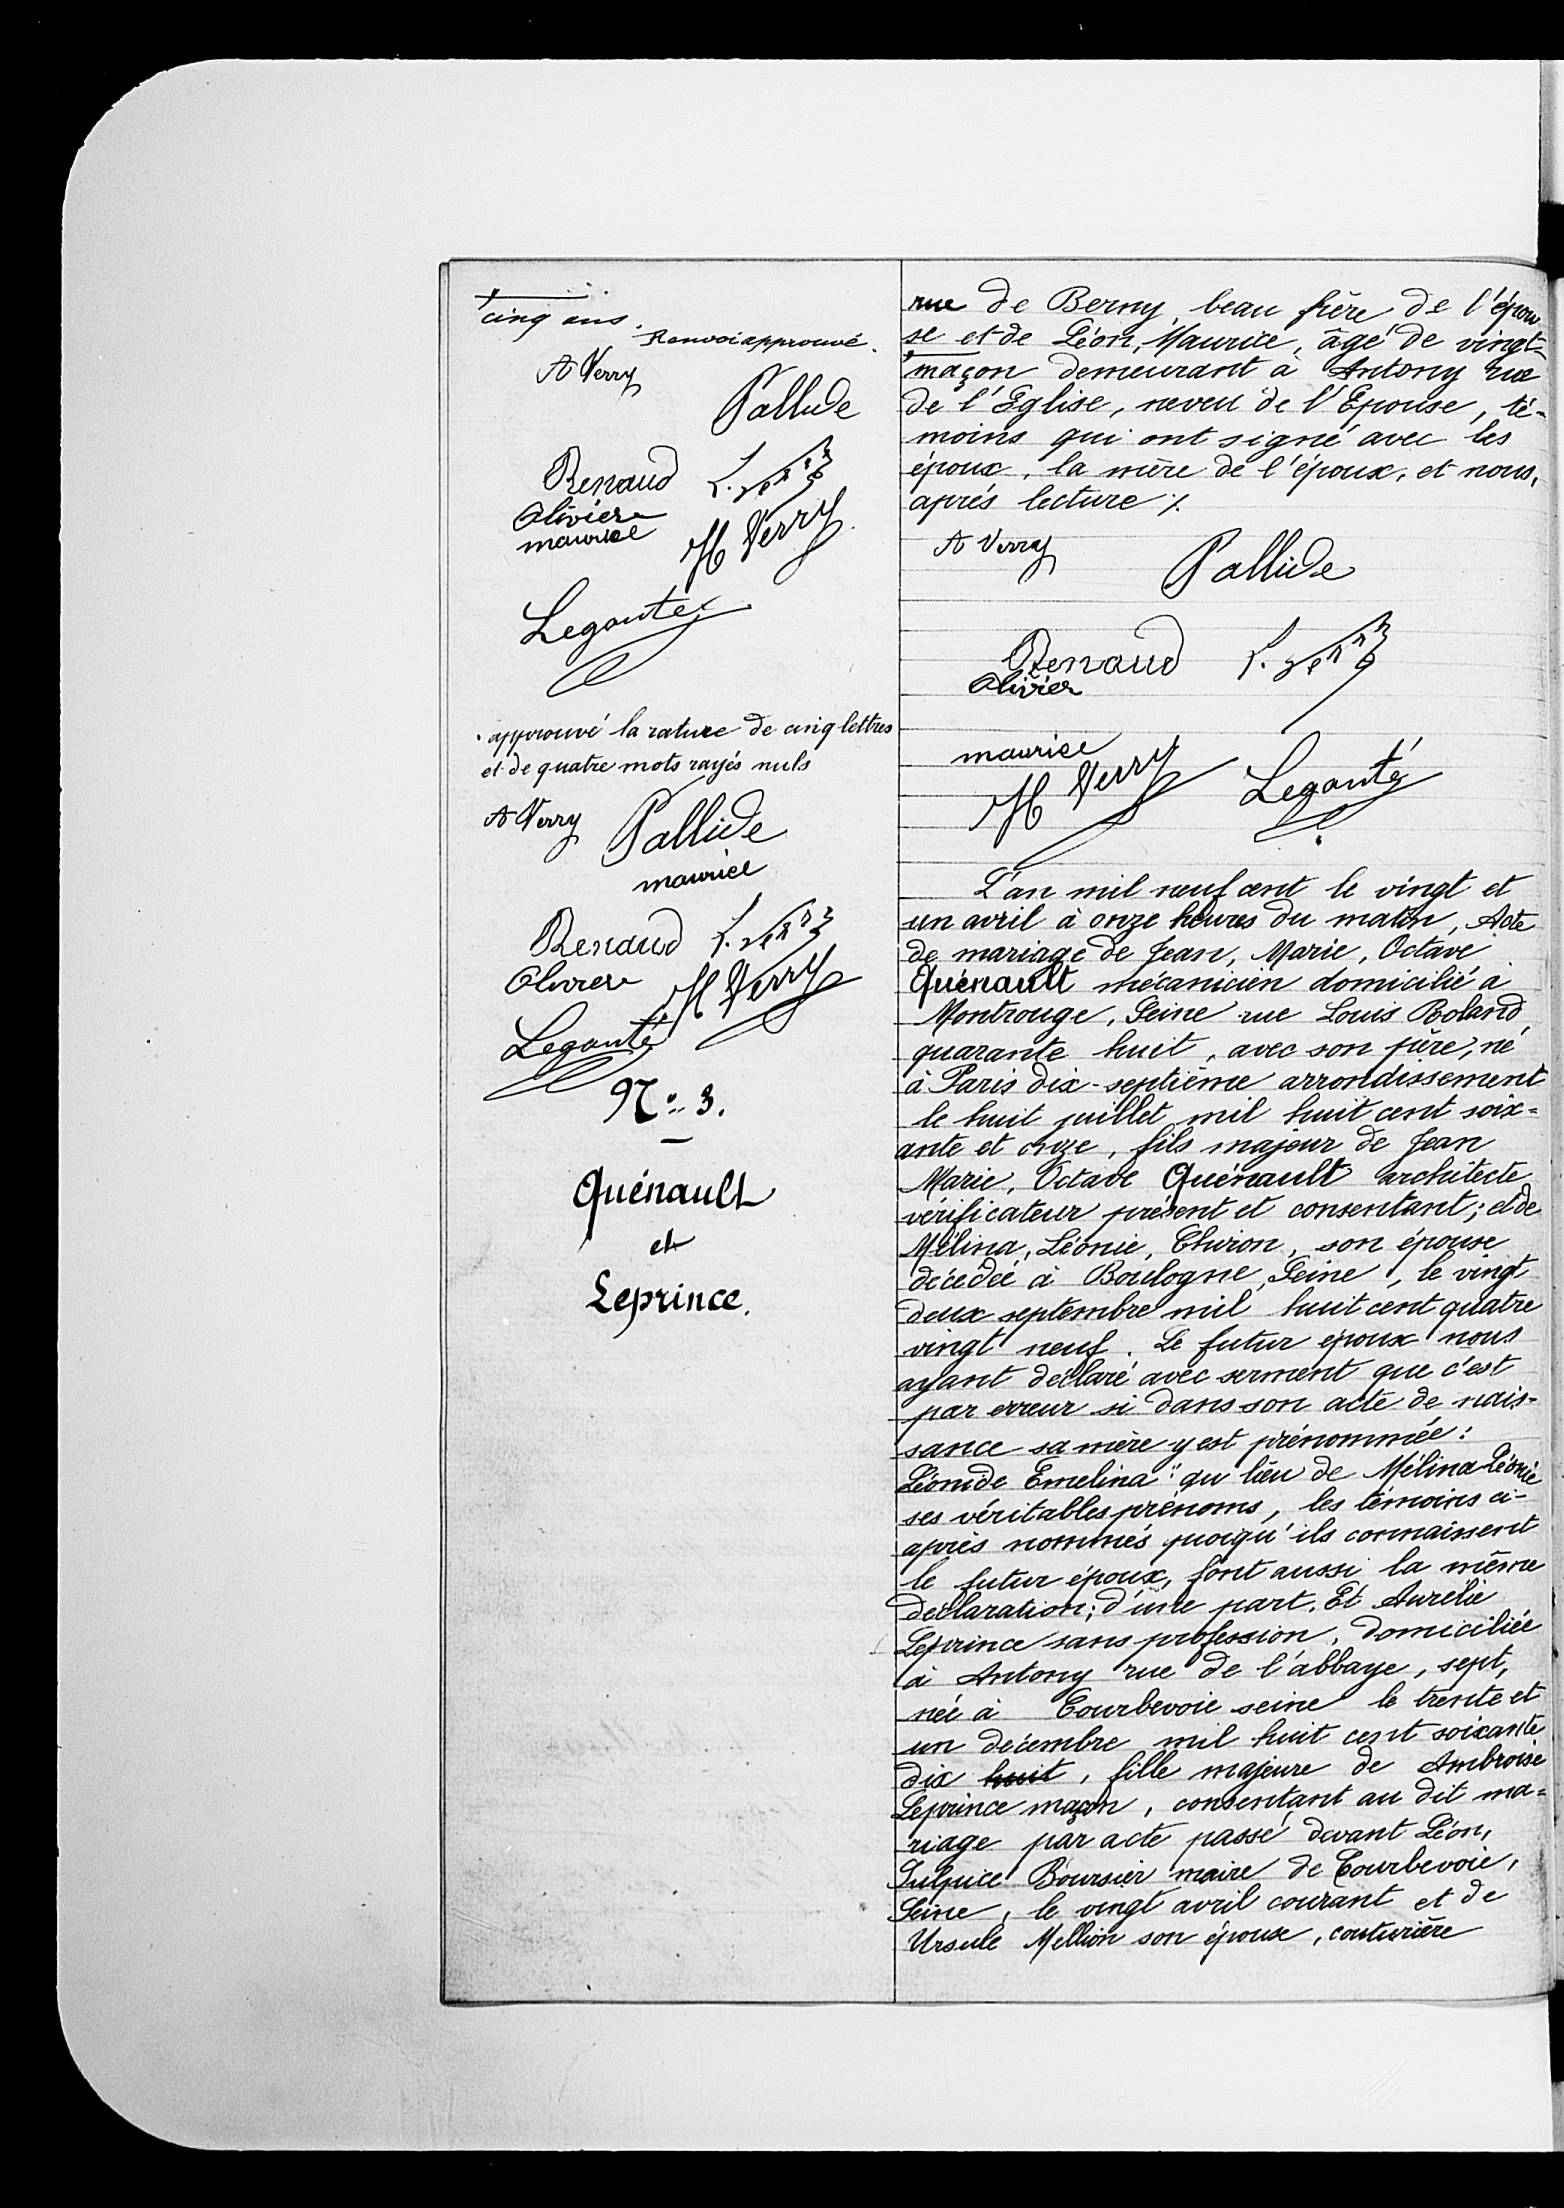rue de Berny, beau père de l'épou
se et de Léon, Maurice, âgé de vingt
maçon demeurant à Antony rue
de l'Eglise, neveu de l'Epouse, té-
moins qui ont signé avec les
époux, la mère de l'époux, et nous,
après lecture ./.
N° 3
Quénault
et
Leprince
L'an mil neuf cent le vingt et
un avril à onze heures du matin, Acte
de mariage de Jean, Marie, Octave
Quénault mécanicien domicilié à
Montrouge, Seine rue Louis Boland
quarante huit, avec son père, né
à Paris dix-septième arrondissement
le huit juillet mil huit cent soix-
ante et onze, fils majeur de Jean
Marie, Octave Quénault architecte
vérificateur présent et consentant; et de
Mélina, Léonie, Chiron, son épouse
décédée à Boulogne, Seine, le vingt
deux septembre mil huit cent quatre
vingt neuf. Le futur époux nous
ayant déclaré avec serment que c'est
par erreur si dans son acte de nais-
sance sa mère y est prénommée:
Léonide Emelina au lieu de Mélina Léonie
ses véritable prénoms, les témoins ci-
après nommés quoiqu'ils connaissent
le futur époux font aussi la même
déclaration, d'une part. Et Aurelie
Leprince sans profession, domiciliée
à Antony rue de l'abbaye, sept
née à Courbevoie seine le trente et
un décembre mil huit cent soixante
dix huit, fille majeure de Ambroise
Leprince maçon, consentant au dit ma-
riage par acte passé devant Léon,
Sulpice Boursier maire de Courbevoie,
Seine le vingt avril courant et de
Ursule Mellion son épouse, couturière
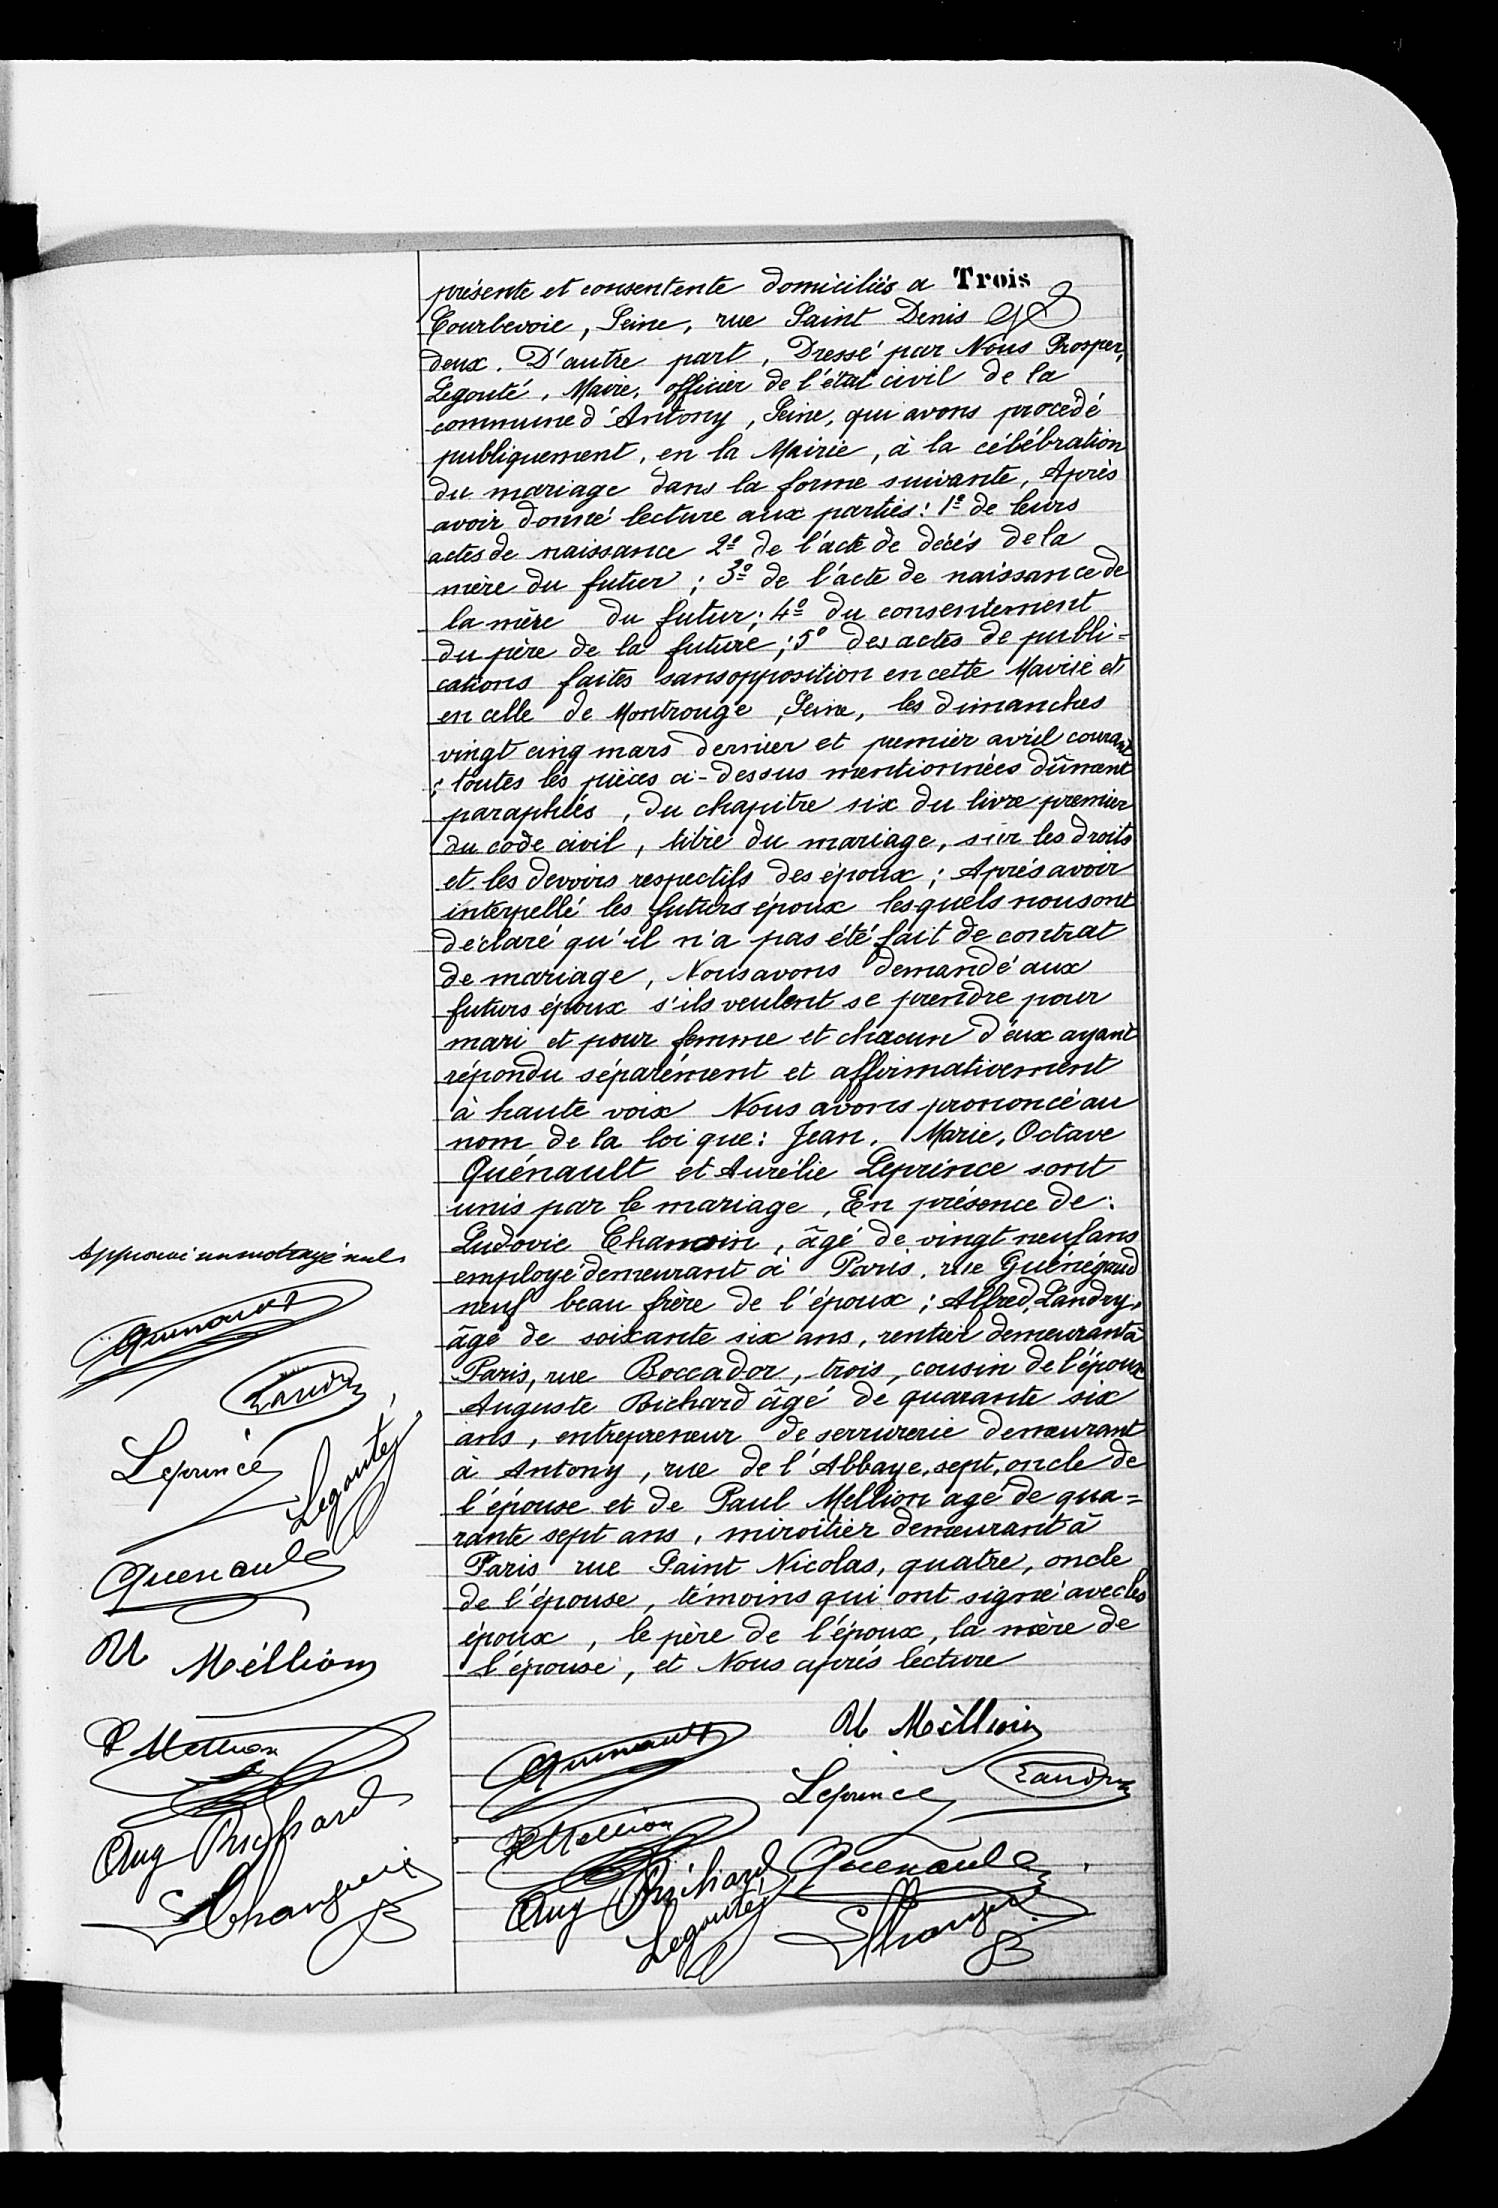présente et consentante domicilié a Trois
Courbevoie, Seine, rue Saint Denis
deux. D'autre part, Dressé par Nous Prosper,
Lecouté, Maire, officier de l'état civil de la
commune d'Antony, Seine, qui avons procédé
publiquement, en la Mairie, à la célébration
du mariage dans la forme suivante, Après
avoir donné lectures aux parties: 1°: de leurs
actes de naissance 2°: de l'acte de décès de la
mère du future: 3°: de l'acte de naissance de
la mère du futur: 4°: du consentement
du père de la future; 5° des actes de publi-
cations faites sans opposition en cette Mairie et
en celle de Montrouge, Seine, les dimanches
vingt cinq mars dernier et premier avril courant
toutes les pièces ci-dessous mentionnées dûmant
paraphés, du chapitre six du livre premier
du code civil, titre du mariage, sur les droits
et les devoirs respectfis des époux: Après avoir
interpellé les futurs époux lesquels nous ont
déclaré qu'il n'a pas été fait de contrat
de mariage, Nous avons demandé aux
futurs époux s'ils veulent se prendre pour
mari et pour femme et chacun d'eux ayant
répondu séparément et affirmativement
à haute voix Nous avons pronnoncé au
nom de la loi que: Jean, Marie, Octave
Quénault et Aurélie Leprince sont
unis par le mariage, En présence de:
Ludovic Chamoin, âgé de vingt neuf ans
employé demeurant à Paris, rue Guégnégaud
neuf beau frère de l'époux; Alfred Landry
âgé de soixante six ans, rentier demeurant à
Paris, rue Boccador, trois, cousin de l'époux
Auguste Richard âgé de quarant six
ans, entrepreneur de serrurerie demeurant
à Antony, rue de l'Abbaye, sept, oncle de
l'épouse et de Paul Mellion agé de qua-
rante sept ans, miroitier demeurant à
Paris rue Saint Nicolas, quatre, oncle
de l'épouse, témoins qui ont signé avec les
époux, le père de l'époux, la mère de
l'épouse, et Nous après lecture